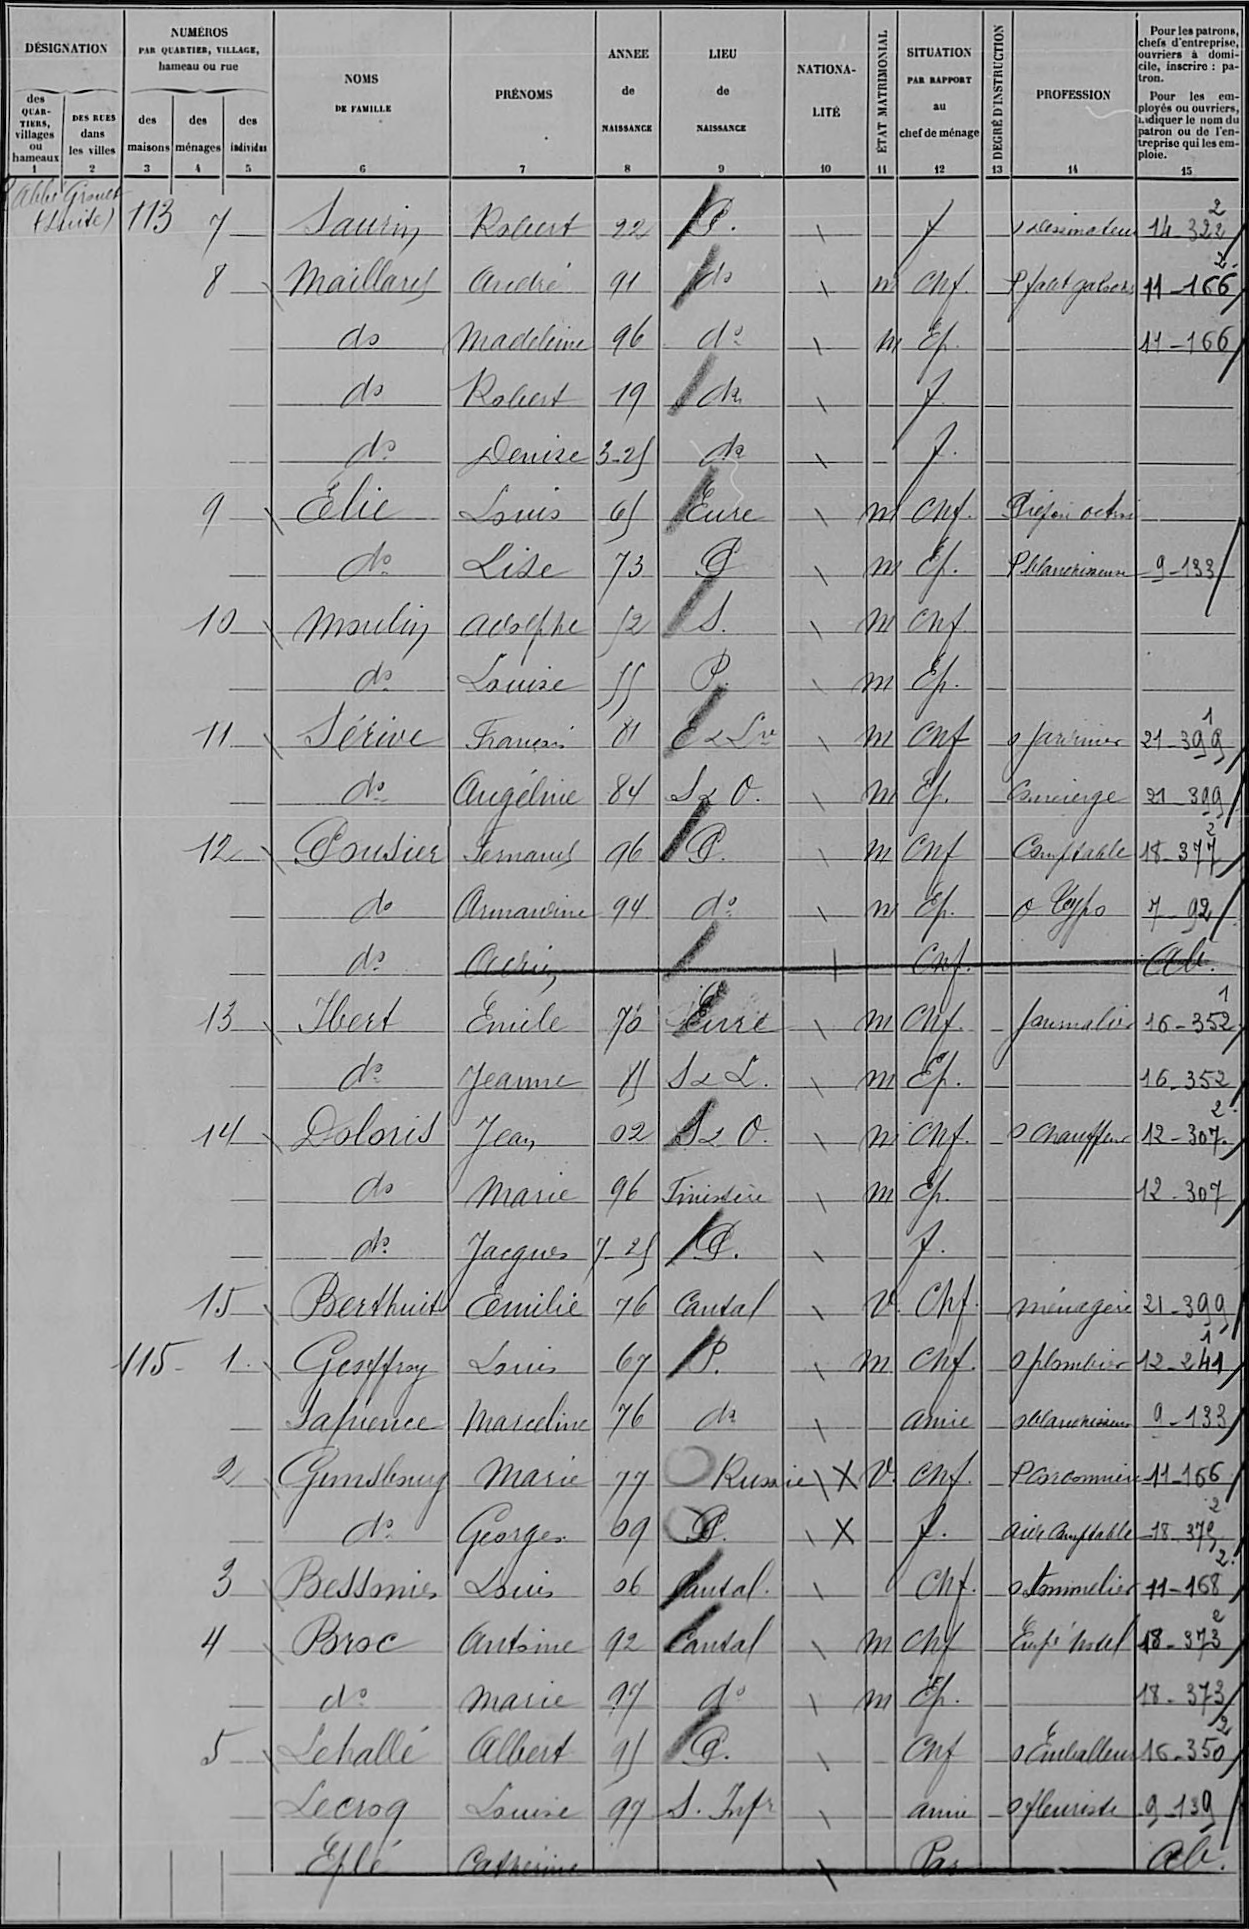Saurin/Robert/22/P /¤/¤/f/¤/o dessinateur/14 322
Maillaud/André/91/d°/¤/m/chf/¤/P fabt galets/11 166
d°/Madeleine/96/d°/¤/m/Ep /¤/¤/11 166
d°/Robert/19/d°/¤/¤/f /¤/¤/¤
d°/Denise/3 21/d°/¤/¤/f /¤/¤/¤
Celie/Louis/61/Eure/¤/m/chf/¤/Prépos octroi/¤
d°/Lise/73/P /¤/m/Ep /¤/Blanchisseuse/9 133
Moulin/Adolphe/52/S /¤/m/Chf /¤/¤/¤
d°/Louise/55/P /¤/m/Ep /¤/¤/¤
Sérive/François/81/E & Lre/¤/m/Chf/¤/o jardinier/21399
d°/Angéline/84/S & O /¤/m/Ep /¤/Concierge/21399
Gousier/Fernand/96/P /¤/m/chf/¤/comptable/18377
d°/Armandine/94/d°/¤/m/Ep /¤/o typo/7 92
d°/Adrien/¤/¤/¤/¤/Chf/¤/¤/Ab
Ibert/Emile/70/Eure/¤/m/Chf/¤/journalier/16 352
d°/Jeanne/81/S & L/¤/m/Ep /¤/¤/16 352
Doloris/Jean/02/S & O/¤/m/Chf/¤/O chauffeur/12 307
d°/Marie/96/Finistère/¤/m/Ep /¤/¤/12307
d°/Jacques/7 25/P /¤/¤/f /¤/¤/¤
Berthuit/Emilie/76/Cantal/¤/V/Chf/¤/ménagère/21 399
Geoffroy/Louis/67/P /¤/m/Chf /¤/o plombier/12 241
Safrienec/Marceline/76/d°/¤/¤/amie/¤/o blanchisseuse/9 133
Gimdburg/Marie/77/Russie/¤/V /Chf /¤/P Cordonniere/11 166
d°/Georges/09/P /¤/¤/f /¤/aide comptable/18 375
Bessonies/Louis/06/Cantal/¤/¤/Chf/¤/o tonnelier/11 168
Broc/Antoine/92/Cantal/¤/m/Chf/¤/Emp Indel/18 373
d°/Marie/99/d°/¤/m/Ep /¤/¤/18 373
Lehallé/Albert/91/P /¤/¤/Chf/¤/o Emballeur/16 350
Lecrocq/Louise/99/S Inf/¤/¤/amie/¤/o fleuriste/9 139
Eflé/Catherine/¤/¤/¤/¤/Par/¤/¤/ab
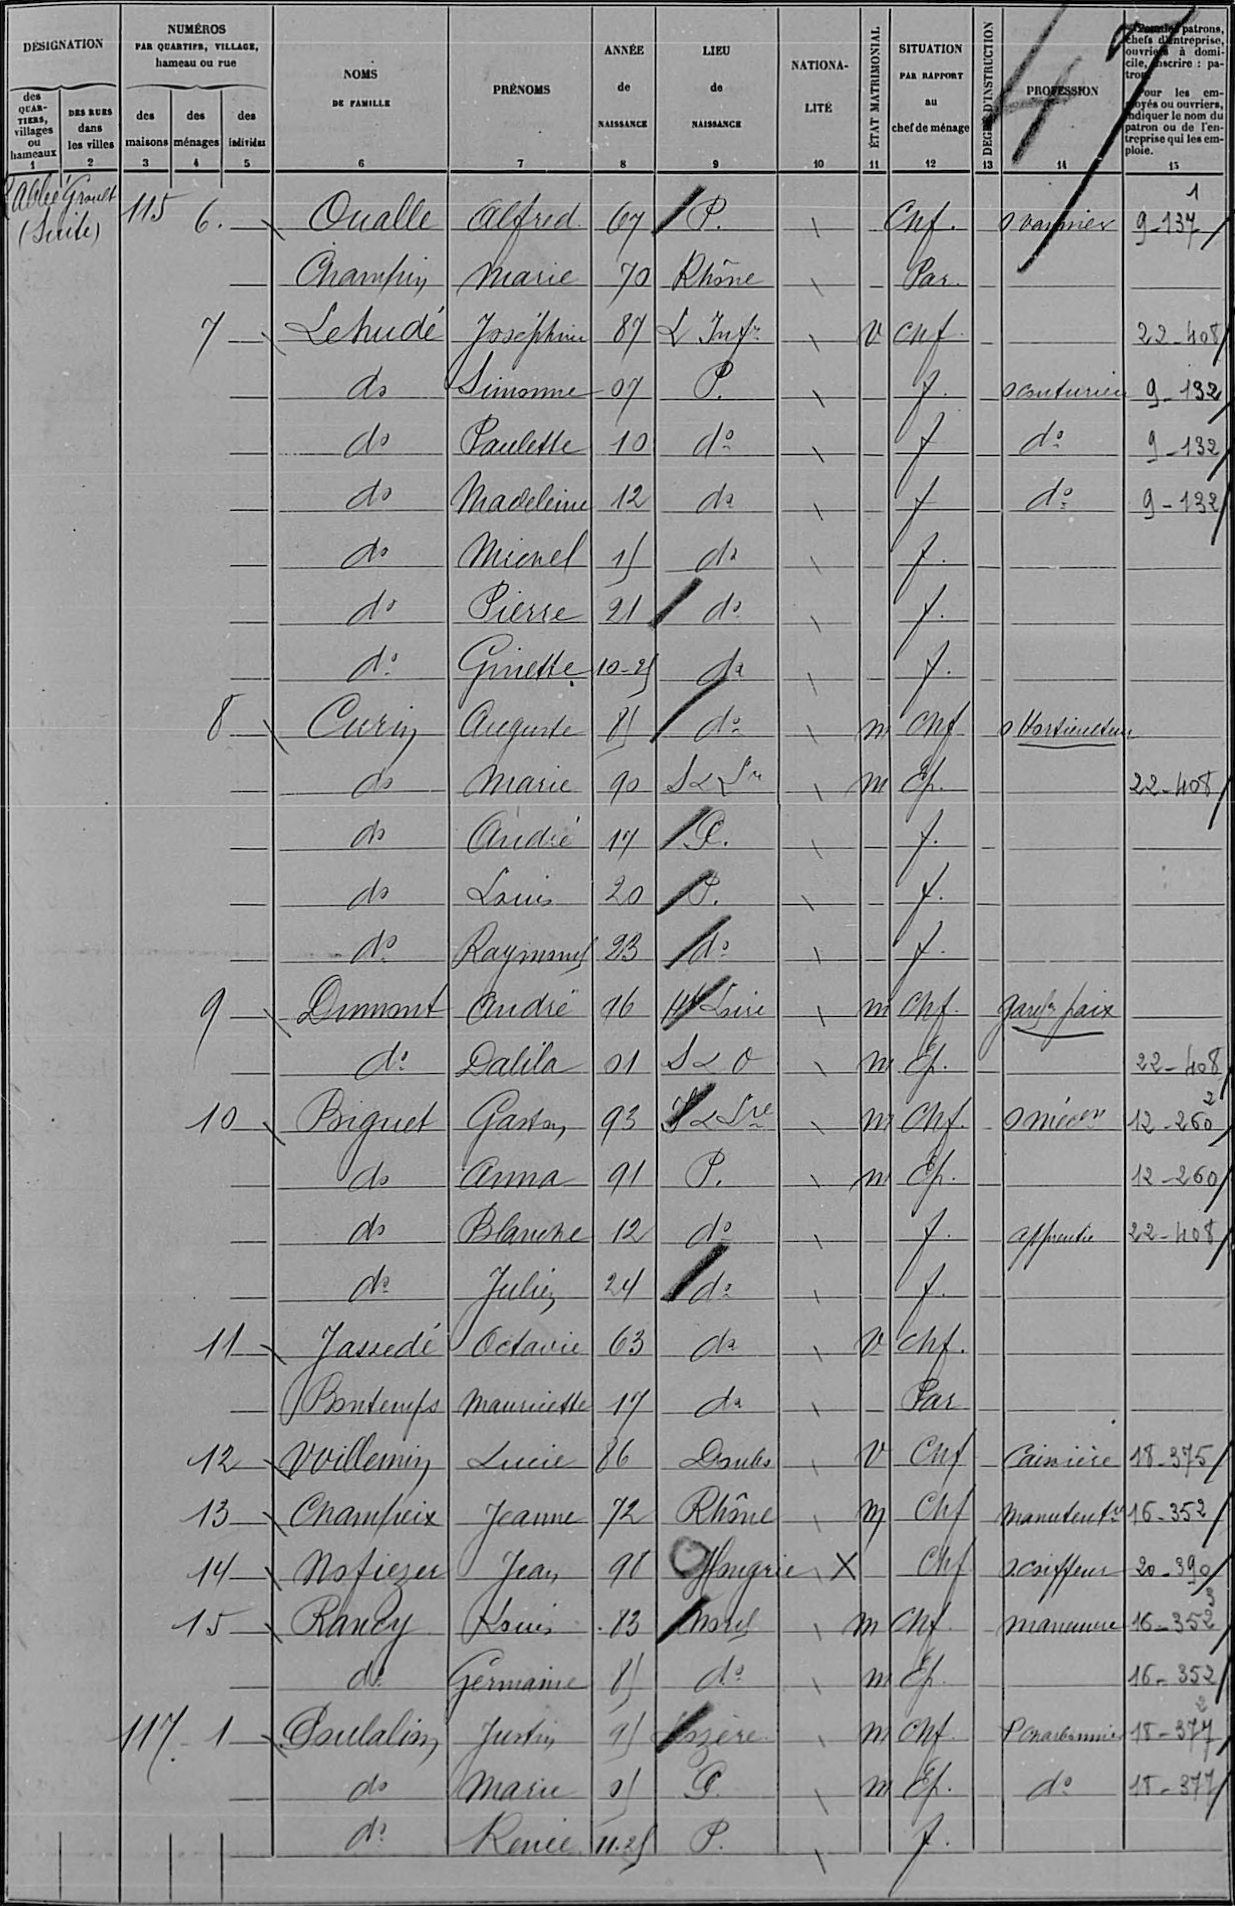Oualle/Alfred/67/P /¤/¤/Chf /¤/o vannier/9 137
Chamfrin/Marie/70/Rhône/¤/¤/Par /¤/¤/¤
Lehudé/Joséphine/87/L Inf/¤/V/Chf/¤/¤/22 408
d°/Simonne/07/P /¤/¤/f /¤/o couturiere/9 132
d°/Paulette/10/d°/¤/¤/f/¤/d°/9 132
d°/Madeleine/12/d°/¤/¤/f/¤/d°/9 132
d°/Michel/15/d°/¤/¤/f /¤/¤/¤
d°/Pierre/21/d°/¤/¤/f /¤/¤/¤
d°/Ginette/10 21/d°/¤/¤/f /¤/¤/¤
Curin/Auguste/81/d°/¤/m/chf/¤/o Horticulture/¤
d°/Marie/90/S&Lre/¤/m/Ep /¤/¤/22 408
d°/André/17/P /¤/¤/f /¤/¤/¤
d°/Louis/20/P /¤/¤/f /¤/¤/¤
d°/Raymond/23/d°/¤/¤/f /¤/¤/¤
Dumont/André/96/Hte Loire/¤/m/chf/¤/Gard paix/¤
d°/Dalila/01/S & O/¤/m/Ep /¤/¤/22408
Biguet/Gaston/93/S & Lre/¤/m/chf/¤/O mécan/12 260
d°/Anna/91/P /¤/m/Ep /¤/¤/12 260
d°/Blanche/12/d°/¤/¤/f /¤/apprentie/22 408
d°/Julie/24/d°/¤/¤/f /¤/¤/¤
Jassedi/Octavie/63/d°/¤/V/chf /¤/¤/¤
Bontemps/mauricette/19/d°/¤/¤/Par/¤/¤/¤
VVillemin/Lucie/86/Doubs/¤/V/Chf/¤/Caissière/18 375
Chamfreix/Jeanne/72/Rhône/¤/m/Chf/¤/manutentre/16 352
Nofiezer/Jean/98/Hongrie/¤/¤/Chf/¤/O coiffeur/20 390
Rancy/Louis/83/Nord/¤/m/Chf/¤/manoeuvre/16 352
d°/Germaine/81/d°/¤/m/Ep /¤/¤/16 352
Poulalion/Justin/91/Lozère/¤/m/chf/¤/P Charbonnier/18 377
d°/Marie/01/P /¤/m/Ep /¤/d°/18 377
d°/Renée/11 25/P /¤/¤/f /¤/¤/¤